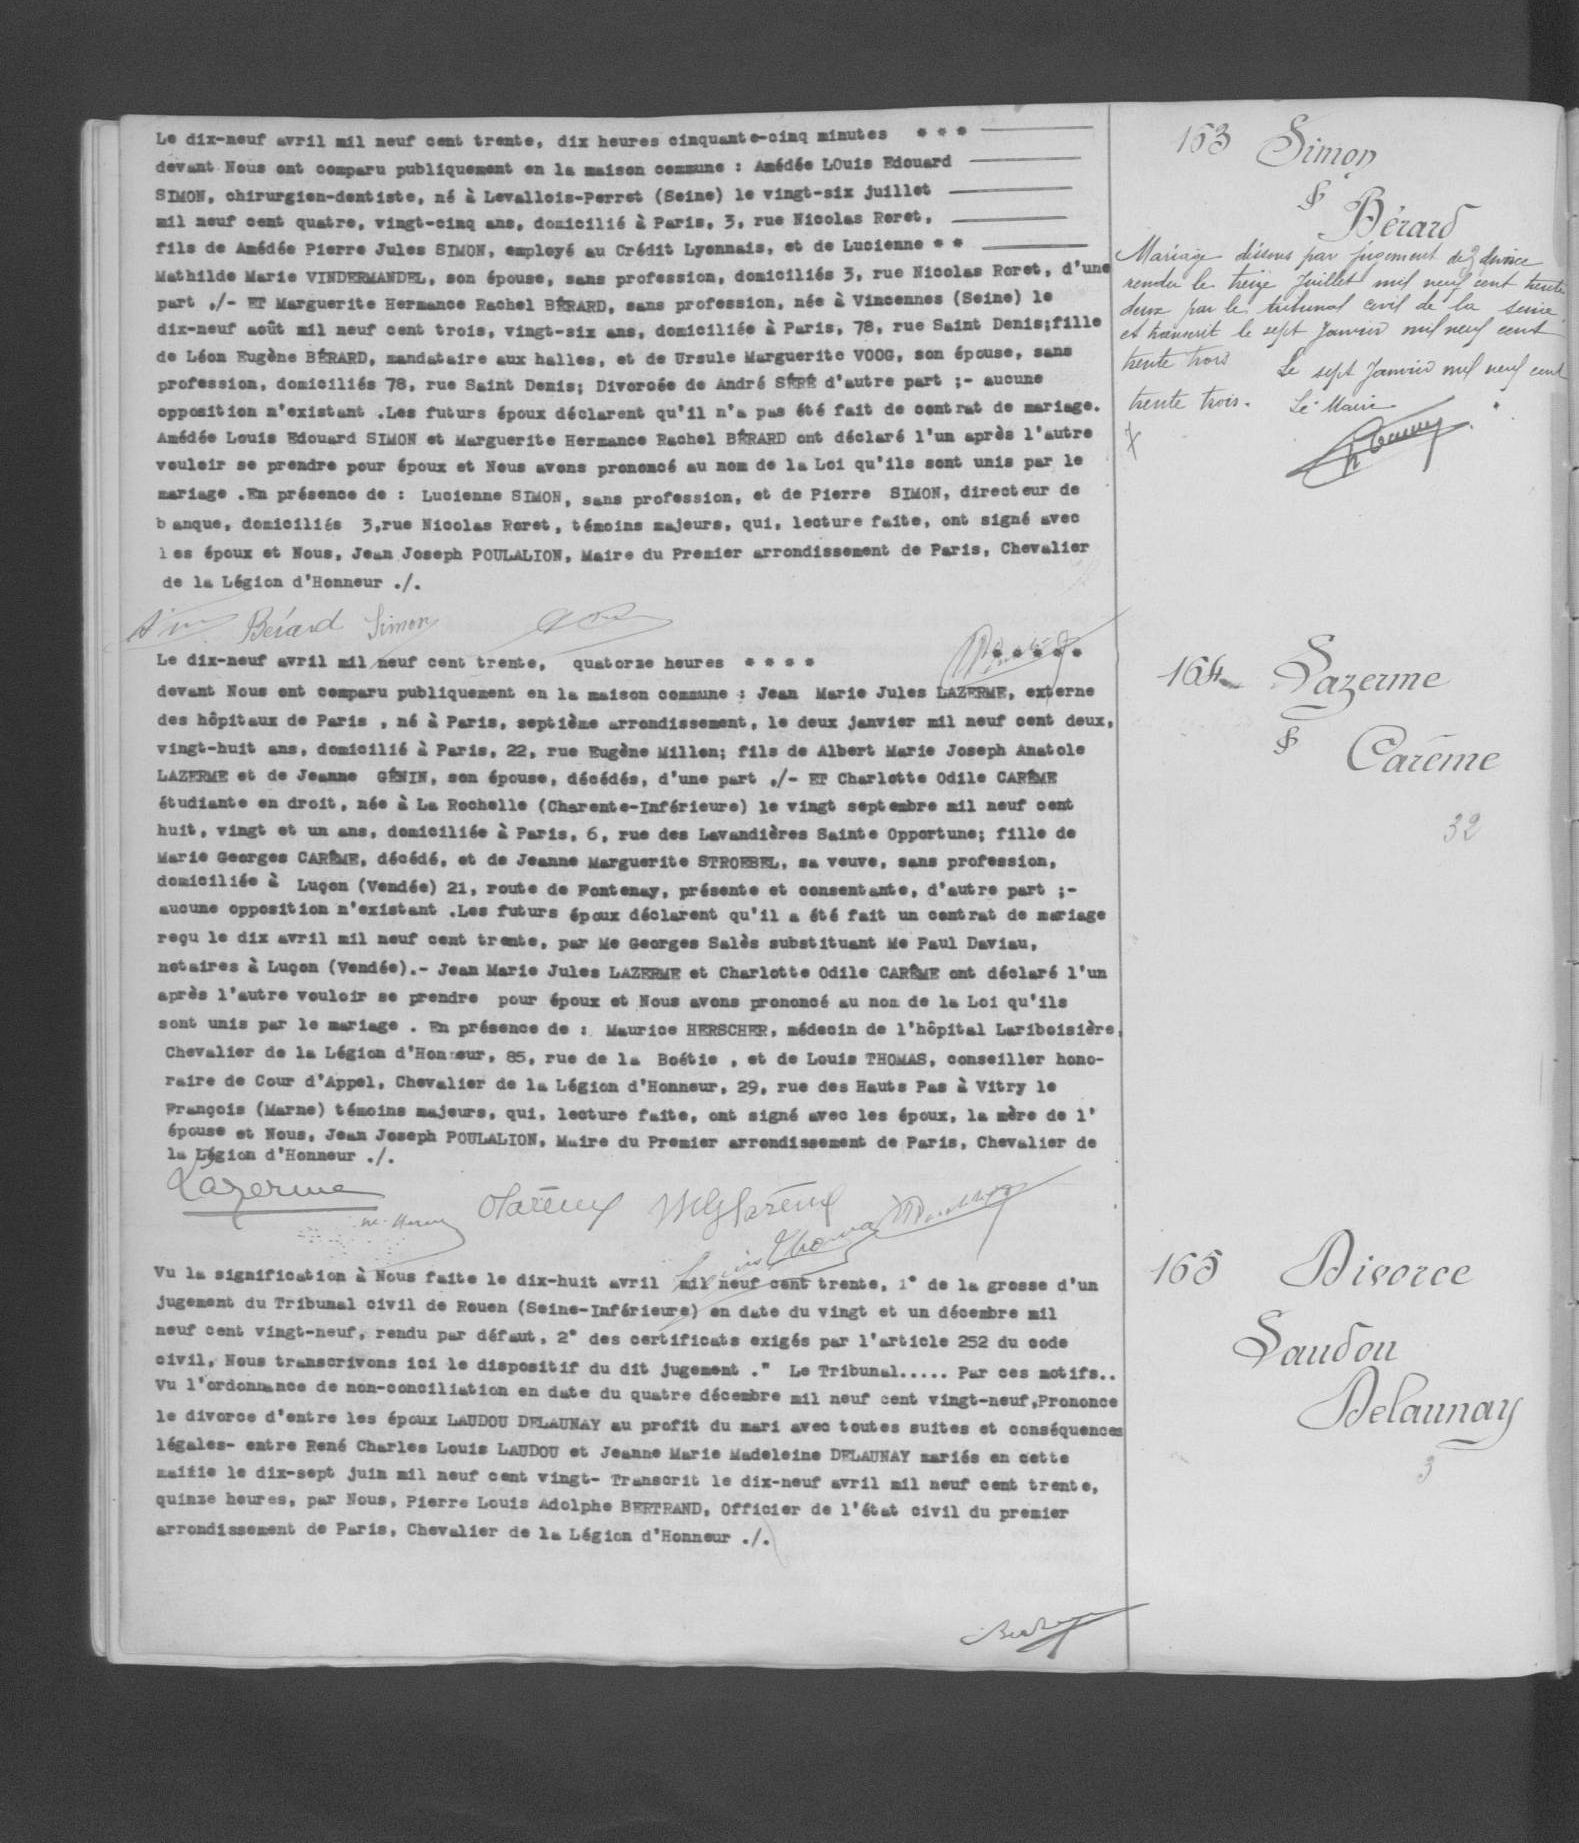Le dix (neuf avril mil neuf cent trente, dix heures cinquante-cinq minutes * * * -
devant Nous ont comparu publiquement en la maison commune: Amédée LOuis Edouard -
SIMON, chirurgien-dentiste, né à Levallois-Perret (Seine) le vingt-six juillet -
mil neuf cent quatre, vingt-cinq ans, domicilié à Paris, 3, rue Nicolas Reret, -
fils de Amédée Pierre Jules SIMON, employé au Crédit Lyonnais, et de Lucienne * * -
Mathilde Marie VINDERMANDEL, son épouse, sans profession, domiciliés 3, rue Nicolas Roret, d'une
part ,/-ET Marguerite Hermance Rachel BERARD, sans profession, née à Vincennes (Seine) le
dix-neuf août mil neuf cent trois, vingt-six ans, domiciliée à Paris, 78, rue Saint Denis; fille
de Léon Eugène BERARD, mandataire aux halles, et de Ursule Marguerite VOOG, son épouse, sans
profession, domiciliés 78, rue Saint Denis; Divorcée de André SERE d'autre part ;-aucune
opposition n'existant. Les futurs époux déclarent qu'il n'a pas été fait de contrat de mariage.
Amédée Louis Edouard SIMON et Marguerite Hermance Rachel BERARD ont déclaré l'un après l'autre
vouloir se prendre pour époux et Nous avons prononcé au nom de la Loi qu'ils sont unis par le
mariage. En présence de: Lucienne SIMON, sans profession, et de Pierre SIMON, directeur de
banque, domiciliés 3, rue Nicolas Reret, témoins majeurs, qui, lecture faite, ont signé avec
les époux et Nous, Jean Joseph POULALION, Maire du Premier arrondissement de Paris, Chevalier
de la Légion d'Honneur ./.
Le dix-neuf avril mil neuf cent trente, quatorze heures ****
devant Nous ont comparu publiquement en la maison commune: Jean Marie Jules LAZERME, externe
des hôpitaux de Paris, né à Paris, septième arrondissement, le deux janvier mil neuf cent deux,
vingt-huit ans, domicilié à Paris, 22, rue Eugène Millen; fils de Albert Marie Joseph Anatole
LAZERME et de Jeanne GENIN, son épouse, décédés, d'une part ,/-ET Charlotte Odile CAREME
étudiante en droit, née à La Rochelle (Charente-Inférieure) le vingt septembre mil neuf cent
huit, vingt et un ans, domiciliée à Paris, 6, rue des Lavandières Sainte Opportune; fille de
Marie Georges CAREME, décédé, et de Jeanne Marguerite STROEBEL, sa veuve, sans profession
domiciliée à Luçon (Vendée) 21, route de Fontenay, présente et consentante, d'autre part ;-
aucune opposition n'existant. Les futurs époux déclarent qu'il a été fait un contrat de mariage
reçu le dix avril mil neuf cent trente, par Me Georges Salès substituant Me Paul Daviau,
notaires à Luçon (Vendée) .-Jean Marie Jules LAZERME et Charlotte Odile CAREME ont déclaré l'un
après l'autre vouloir se prendre pour époux et Nous avons prononcé au nom de la Loi qu'ils
sont unis par le mariage. En présence de: Maurice HERSCHER, médecin de l'hôpital Lariboisière,
Chevalier de la Légion d'Honneur, 85, rue de la Boétie, et de Louis THOMAS, conseiller hono-
raire de Cour d'Appel, Chevalier de la Légion d'Honneur, 29, rue des Hauts Pas à Vitry le
François (Marne) témoins majeurs, qui, lecture faite, ont signé avec les époux, la mère de l'
épouse et nous, Jean Joseph POULALION, Maire du Premier arrondisement de Paris, Chevalier
de la Légion d'Honneur ./.
Vu la signification à Nous faite le dix-huit avril mil neuf cent trente, 1° de la grosse d'un
jugement du Tribunal civil de Rouen (Seine-Inférieure) en date du vingt et un décembre mil
neuf cent vingt-neuf, rendu par défaut, 2° des certificats exigés par l'article 252 du code
civil, Nous transcrivons ici le dispositif du dit jugement." Le Tribunal..... Par ces motifs ..
Vu l'ordonnance de non-conciliation en date du quatre décembre mil neuf cent vingt-neuf, Prononce
le divorce d'entre les époux LAUDOU DELAUNAY au profit du mari avec toutes suites et conséquences
légales-entre René Charles Louis LAUDOU et Jeanne Marie Madeleine DELAUNAY mariés en cette
mairie le dix-sept juin mil neuf cent vingt-Transcrit le dix-neuf avril mil neuf cent trente,
quinze heures, par Nous, Pierre Louis Adolphe BERTRAND, Officier de l'état civil du premier
arrondissement de Paris, Chevalier de la Légion d'Honneur ./.
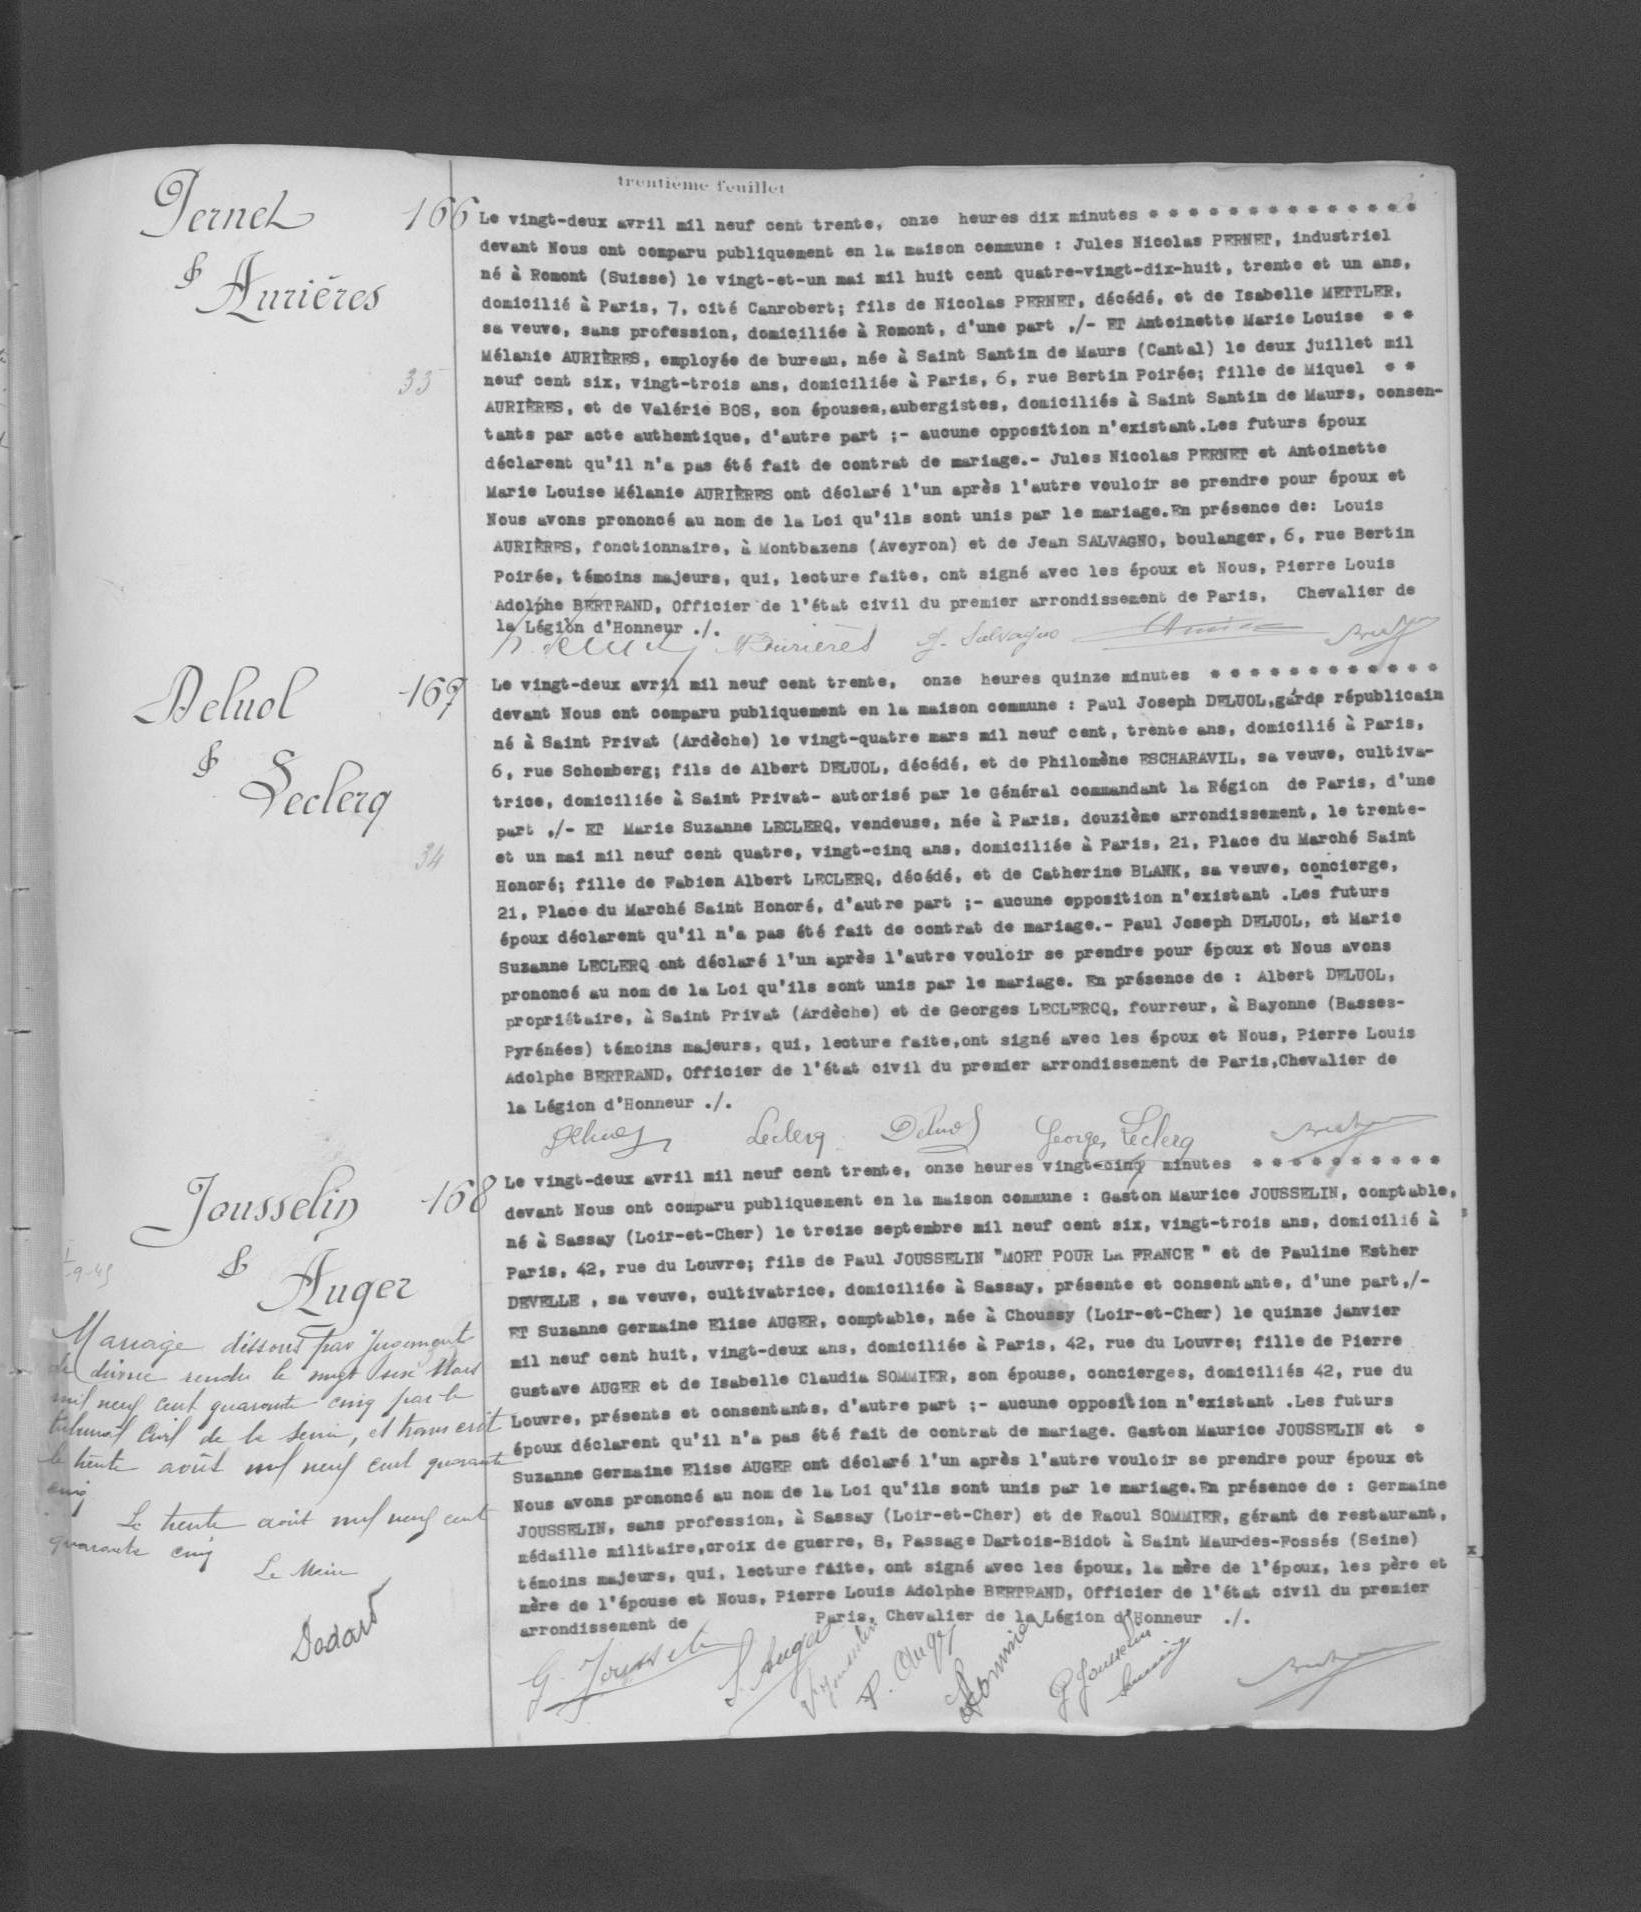Le vingt-deux avril mil neuf cent trente, onze heures dix minutes ****
devant Nous ont comparu publiquement en la maison commune: Jules Nicolas PERNET, industriel
né à Romont (Suisse) le vingt-et-un mai mil huit cent quatre-vingt-dix-hiut, trente et un ans,
domicilié à Paris, 7, cité Canrobert; fils de Nicolas Pernet, décédé, et de Isabelle METTLER,
sa veuve, sans profession, domiciliée à Romont, d'une part ,/-ET Antoinette Marie Louise * *
Mélanie AURIERES, employée de bureau, née à Saint Santin de Maurs (Cantal) le deux juillet mil
neuf cent six, vingt-trois ans, domiciliée à Paris, 6, rue Bertin Poirée; fille de Miquel * *
AURIERES, et de Valérie BOS, son épouse *, aubergistes, domiciliés à Saint Santin de Maurs, consen-
tants par acte authentique, d'autre part ;-aucune opposition n'existat. Les futurs époux
déclarent qu'il n'a pas été fait de contrat de mariage .-Jules Nicolas PERNET et Antoinette
Marie Louise Mélanie AURIERES ont déclaré l'un après l'autre vouloir se prendre pour époux et
Nous avons prononcé au nom de la Loi qu'ils sont unis par le mariage. En présence de: Louis
AURIERES, fonctionnaire, à Montbazens (Aveyron) et de Jean SALVAGNO, boulanger, 6, rue Bertin
Poirée, témoins majeurs, qui, lecture faite, ont signé avec les époux et Nous, Pierre Louis
Adolphe BERTRAND, Officier de l'état civil du premier arrondisement de Paris, Chevalier de
la Légion d'Honneur ./.
Le vingt-deux avril mil neuf cent trente, onze heures quinze minutes ****
devant Nous ont comparu publiquement en la maison commune: Paul Joseph DELUOL, garde républicain
né à Saint Privat (Ardèche) le vingt-quatre mars mil neuf cent, trente ans, domicilié à Paris,
6, rue Schonberg; fils de Albert DELUOL, décédé, et de Philomène ESCHARAVIL, sa veuve, cultiva-
trice, domiciliée à Saint Privat-autorisé par le Général commandant la Région de Paris, d'une
part ;/-ET Marie Suzanne LECLERQ, vendeuse, née à Paris, douzième arrondissement, le trente-
et un mai mil neuf cent quatre, vingt-cinq ans, domiciliée à Paris, 21, Place du Marché Saint
Honoré; fille de Fabien Albert LECLERQ, décédé, et de Catherine BLANK, sa veuve, concierge,
21, Place du Marché Saint Honoré, d'autre part ;-aucune opposition n'existant. Les futurs
époux déclarent qu'il n'a pas été fait de contrat de mariage .-Paul Joseph DELUOL, et Marie
Suzanne LECLERQ ont déclaré l'un après l'autre vouloir se prendre pour époux et Nous avons
prononcé au nom de la Loi qu'ils sont unis par le mariage. En présence de: Albert DELUOL,
propriétaire, à Saint Privat (Ardèche) et de Georges LECLERQ, fourreur, à Bayonne (Basses-
Pyrénées) témoins majeurs, qui, lecture faite, ont signé avec les époux et Nous, Pierre Louis
Adolphe BERTRAND, Officier de l'état civil du premier arrondissement de Paris, Chevalier de
la Légion d'Honneur ./.
Le vingt-deux avril mil neuf cent trente, onze heures vingt-cinq minutes ****
devant Nous ont comparu publiquement en la maison commune: Gaston Maurice JOUSSELIN, comptable
né à Sassay (Loir-et-Cher) le treize septembre mil neuf cent six, vingt-trois ans, domicilié à
Paris, 42, rue du Louvre; fils de Paul JOUSSELIN "MORT POUR LA FRANCE" et de Pauline Esther
DEVELLE, sa veuve, cultivatrice, domiciliée à Sassay, présente et consentante, d'une part ,/-
ET Suzanne Germaine Elise AUGER, comptable, née à Choussy (Loir-et-Cher) le quinze janvier
mil neuf cent huit, vingt-deux ans, domiciliée à Paris, 42, rue du Louvre; fille de Pierre
Gustave AUGER et de Isabelle Claudia SOMMIER, son épouse, concierges, domiciliés 42, rue du
Louvre, présents et consentants, d'autre part ;-aucune opposition n'existant. Les futurs
époux déclarent qu'il n'a pas été fait de contrat de mariage. Gaston Maurice JOUSSELIN et *
Suzanne Germaine Elise AUGER ont déclaré l'un après l'autre vouloir se prendre pour époux et
Nous avons prononcé au nom de la Loi qu'ils sont unis par le mariage. En présence de: Germaine
JOUSSELIN, sans profession, à Sassay (Loir-et-Cher) et de Raoul SOMMIER, gérant de restaurant,
médaille militaire, croix de guerre, 8, Passage Dartois-Bidot à Saint Maur-des-Fossés (Seine)
témoins majeurs, qui, lecture faite, ont signé avec les époux, la mère de l'époux, les père et
mère de l'épouse et Nous, Pierre Lous Adolphe BERTRAND, Officier de l'état civil du premier
arrondissement de Paris, Chevalier de la Légion d'Honneur ./.